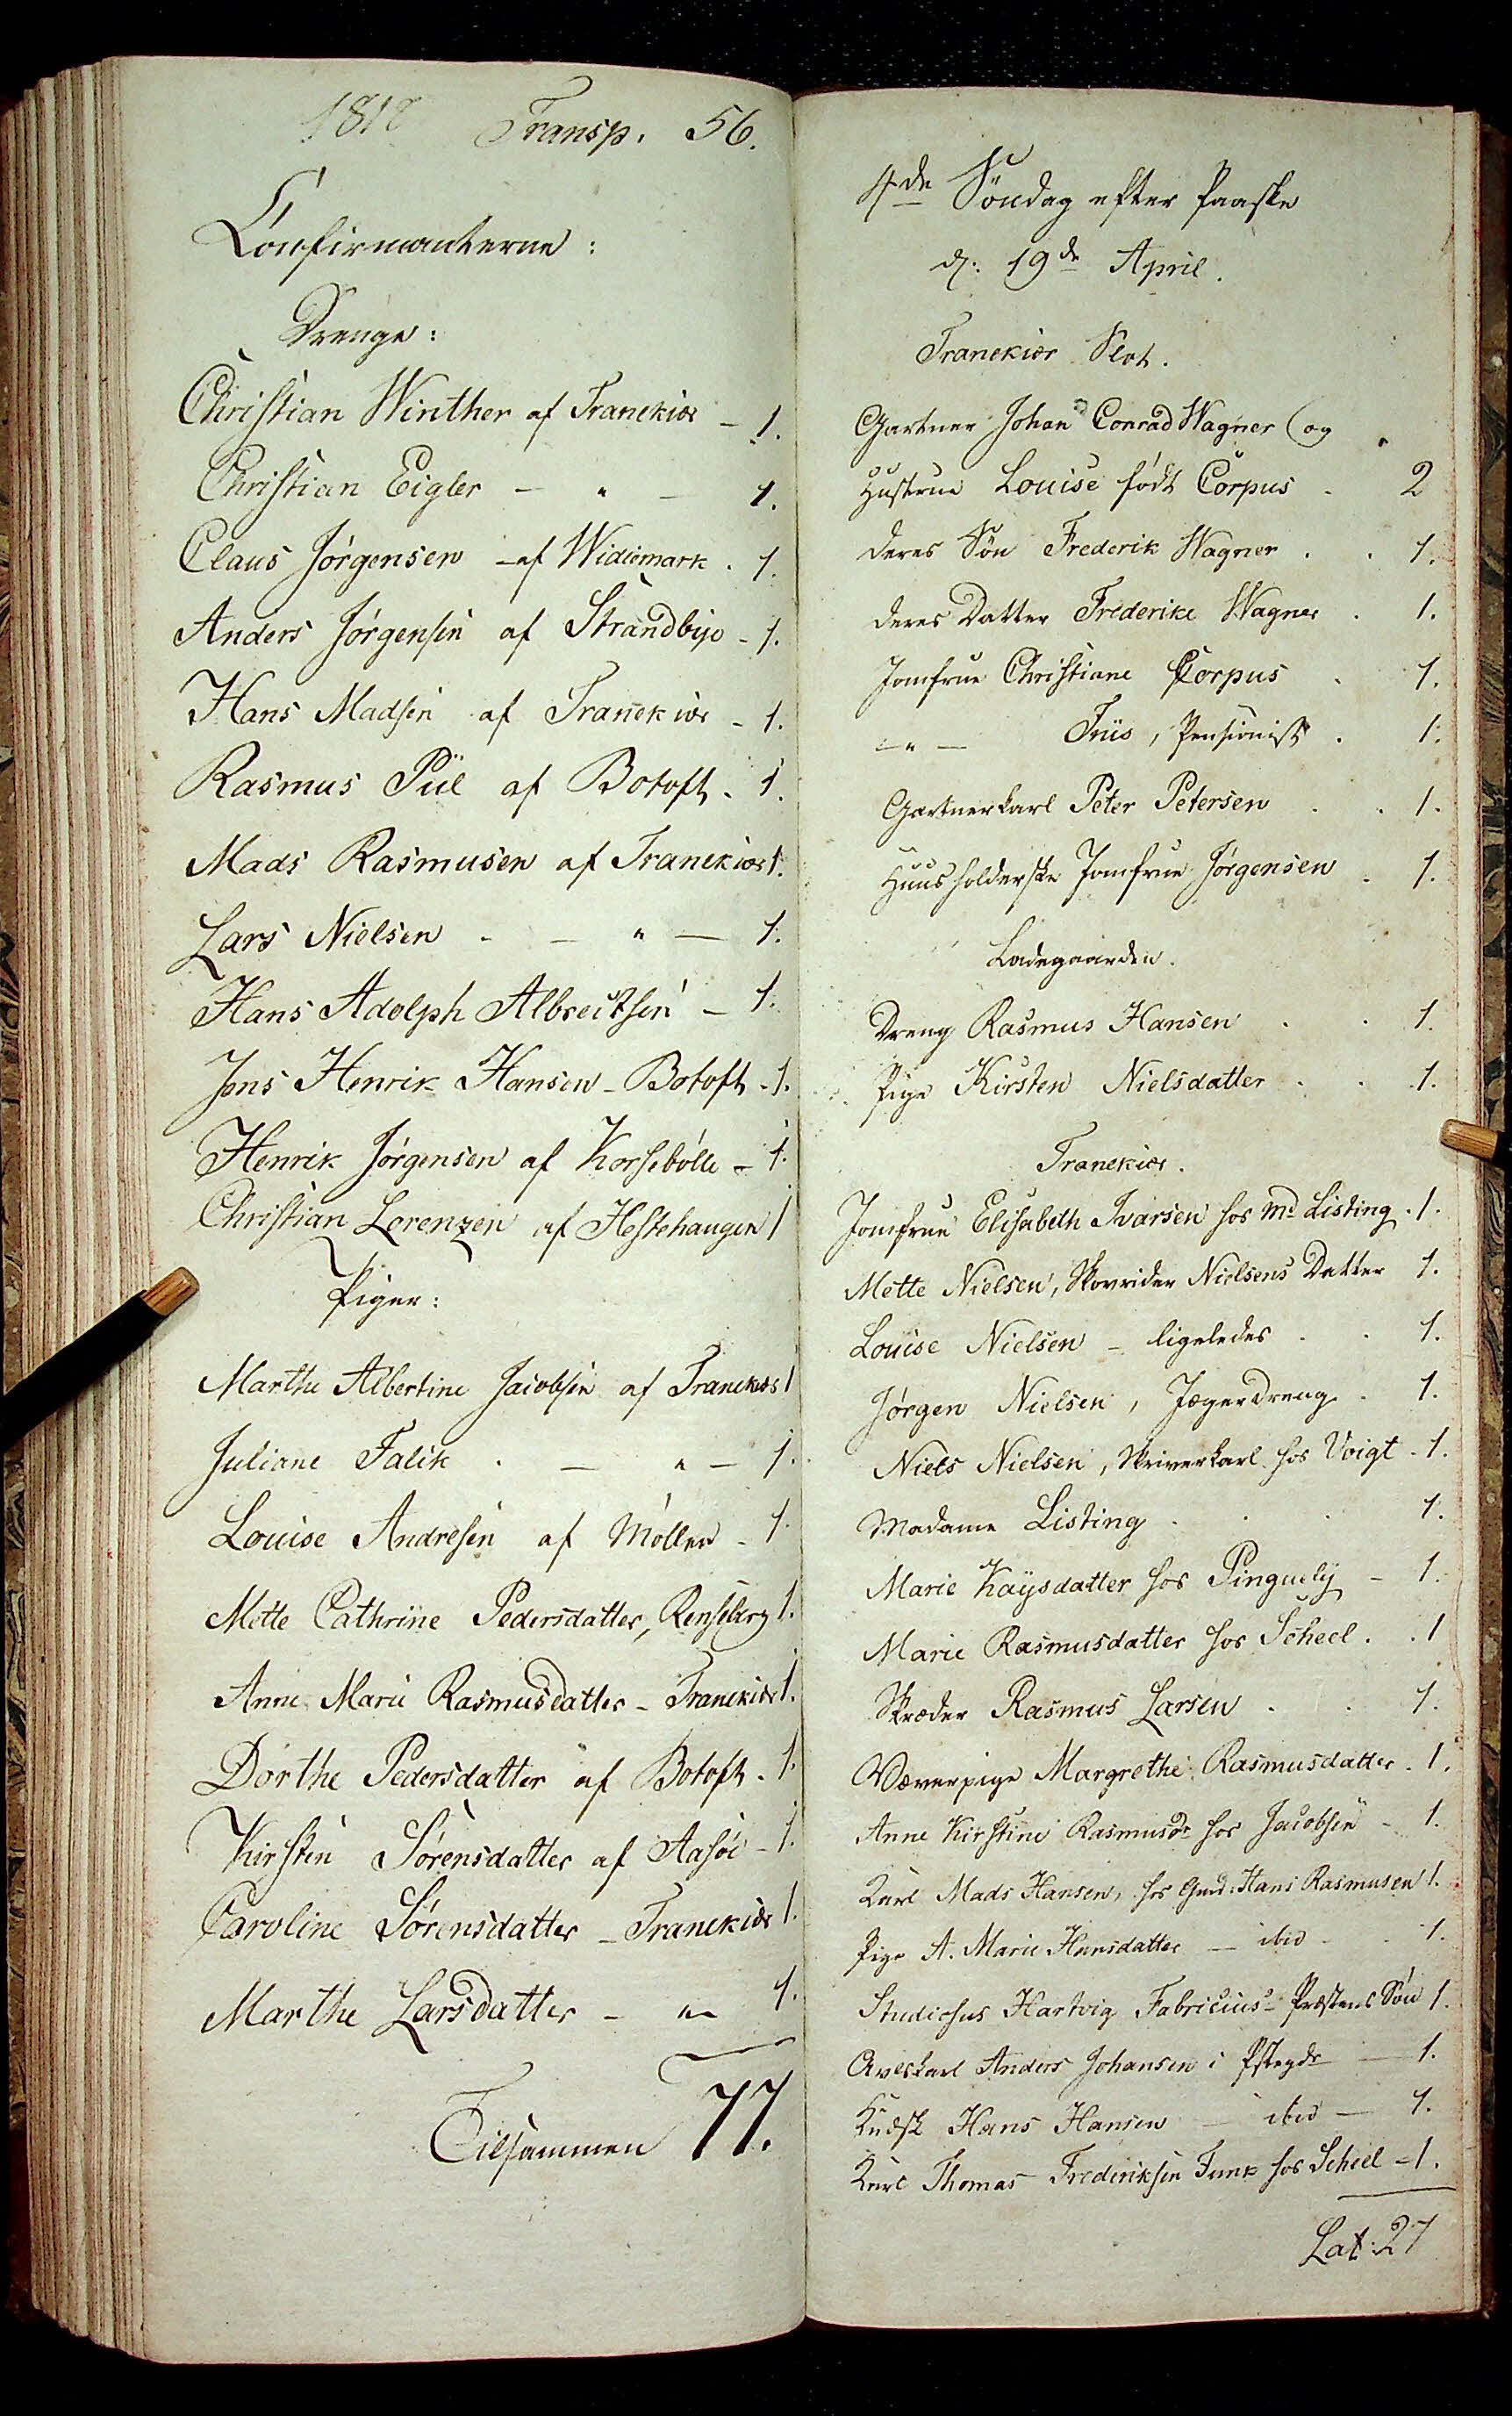1818
Transp. 56.
Confirmanterne:
Drenge:
Christian Wirther af Tranekiær - 1.
Christian Eigler -"- 1.
Claus Jørgensen af Widiemark . 1.
Anders Jørgensen af Strandbye - 1.
Hans Madsen af Tranekiær - 1.
Rasmus Piil af Botoft . 1.
Mads Rasmusen af Tranekiær 1.
Lars Nielsen - -"- 1.
Hans Adolph Albrectsen - 1.
Jens Henrik Hansens - Botoft . 1.
Henrik Jørgensen af Korsebølle - 1.
Christian Sørensen af Hestehaugen 1
Piger:
Marthe Albertine Jacobsen af Tranekiær 1
Juliane Falck . -"- 1.
Louise Andersen af Møllen - 1
Mette Cathrine Pedersdatter, Renseberg 1.
Anne Marie Rasmusdatter - Tranekiær 1.
Dorthe Pedersdatter af Botoft . 1.
Kirsten Sørensdatter af Aasøe - 1.
Caroline Sørensdatter - Tranekiær 1.
Marthe Larsdatter -"- 1.
Tilsammen 77.
4de Søndag efter Paaske
d: 19de April.
Tranekiær Slot.
Gartner, Johan Conrad Wagner og
Hustrue Louise født Corpus . 2
deres Søn Frederik Wagner . . 1.
deres Datter Friderike Wagner . 1.
Jomfrue Christiane Corpus . 1.
-"- Friis Pensionist . 1.
Gartnerkarl Peter Petersen . . 1.
Huusholderse Jomfrue Jørgensen . 1.
Ladegaarden.
Dreng Rasmus Hansen . . 1.
Pige Kirsten Nielsdatter . . . 1.
Tranekiær.
Jomfrue Elisabeth Ivarsen hos Md Listing . 1.
Mette Nielsen, Skovrider Nielsens Datter 1.
Louise Nielsen - ligeledes . . 1.
Jørgen Nielsen, Jægerdreng . 1.
Niels Nielsen, Skriverkarl hos Voigt . 1.
Madame Listing . . . 1.
Marie Kaysdatter hos Pinguely - 1.
Marie Rasmusdatter hos Scheel . . 1
Skræder Rasmus Larsen . . 1.
Væverpige, Margrethe Rasmusdatter . 1.
Anne Kirstine Rasmusdr hos Jacobsen - 1.
Karl Mads Hansen, hos Grd: Hans Rasmusen 1.
Pige A. Marie Hansdatter - ibid . . 1.
Studiosus Hartvig Fabricius - Præstens Søn 1.
Avlskarl Anders Johansen i Pstegdr - 1.
Kudsk Hans Hansen - ibid - 1.
Karl Thomas Frideriksen Funk hos Scheel
Lat: 27
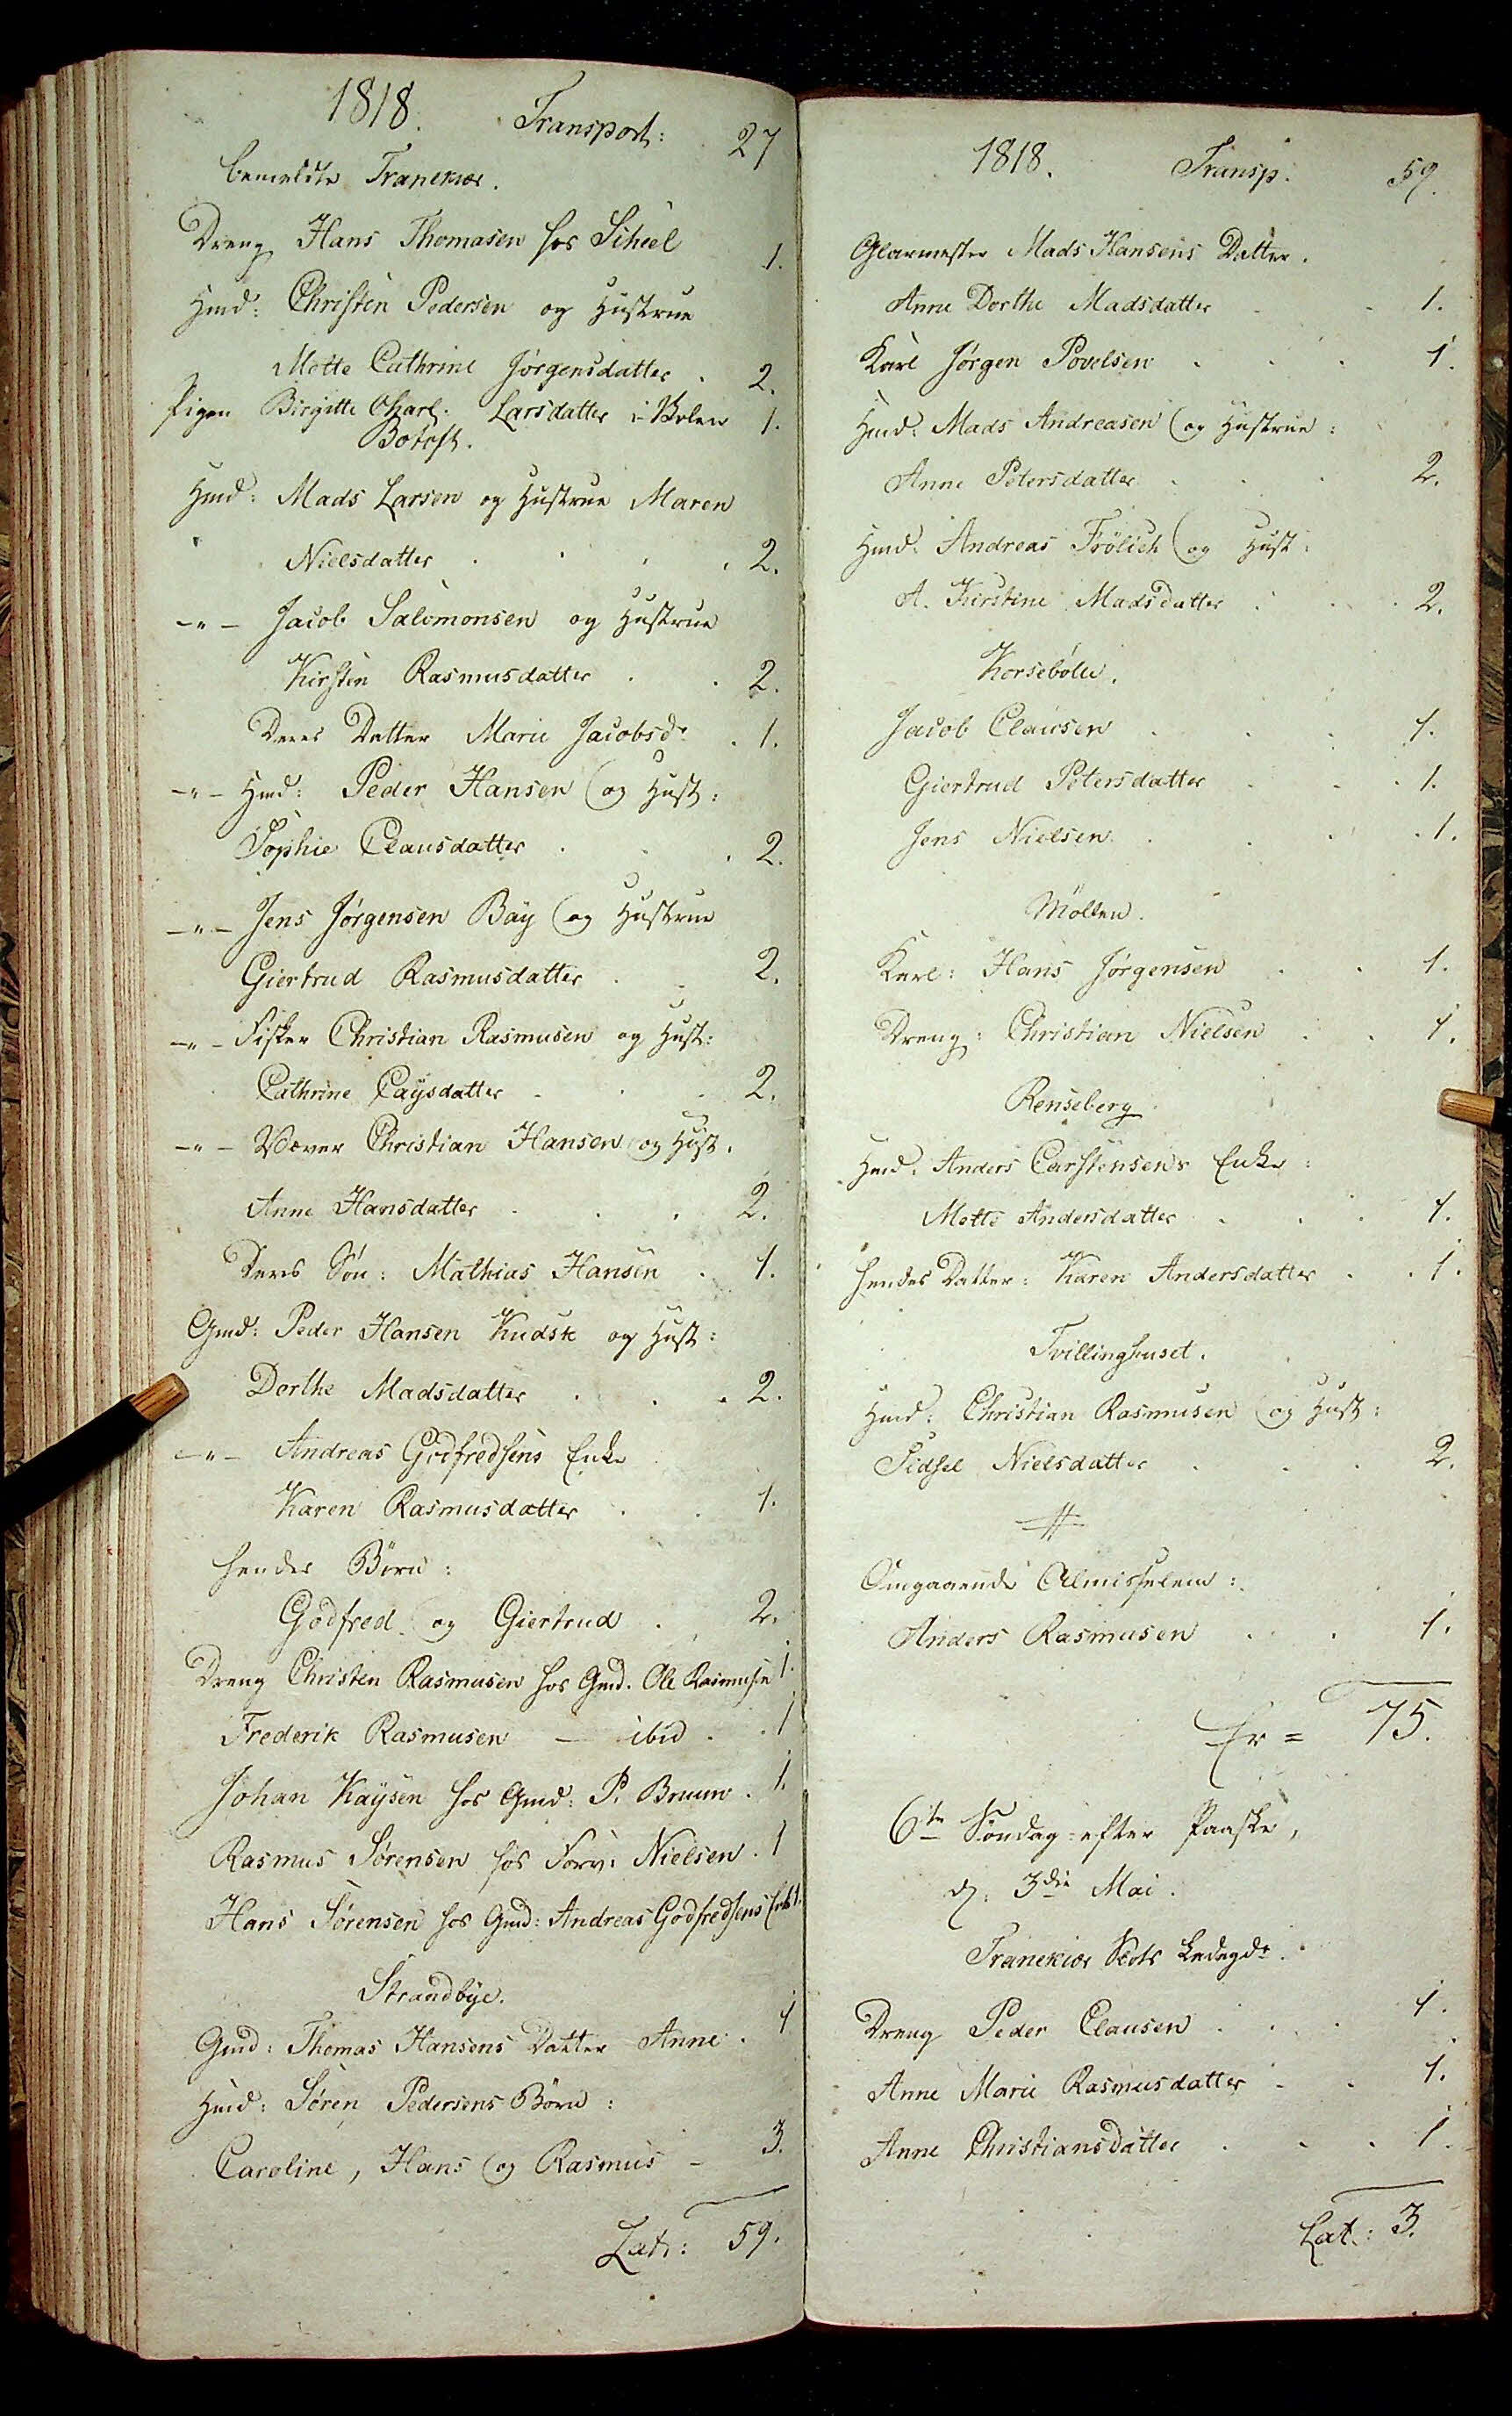1818. . Transport: 27.
bemeldte Tranekiær.
Dreng Hans Thomasen hos Scheel 1.
Hmd: Christen Pedersen og Hustrue
Mette Cathrine Jørgensdatter . 2.
Pigen Birgitte Charl. Larsdatter i Skolen 1.
Botoft.
Hmd: Mads Larsen og Hustrue Maren
Nielsdatter . . . . 2.
-"- Jacob Salomonsen og Hustrue
Kirsten Rasmusdatter . . 2.
Deres Datter Marie Jacobsdr . 1.
-"- Hmd: Peder Hansen og Hust:
Sophie Clausdatter . . . 2.
-"- Jens Jørgensen Bay og Hustrue
Giertrud Rasmusdatter . . 2.
-"- Fisker Christian Rasmusen og Hustr:
Cathrine Caysdatter . . . 2.
-"- Væver Christian Hansen og Hust:
Anne Hansdatter . . . 2.
Deres Søn: Mathias Hansen . 1.
Gmd: Peder Hansen Kudsk og Hust:
Dorthe Madsdatter . . . 2.
-"- Andreas Godfredsens Enke
Karen Rasmusdatter . . 1.
hendes Børn:
Godfred og Giertrud . 2.
Dreng Christen Rasmusen hos Gmd. Ole Rasmusen 1.
Frederik Rasmusen - ibid . .1
Johan Kaysen hos Gmd: P. Bruun . 1
Rasmus Sørensen hos Forv: Nielsen . 1
Hans Sørensen hos Gmd: Andreas Godfredsens Enke 1
Strandbye.
Gmd: Thomas Hansens Datter Anne . 1
Hmd: Søren Pedersens Børn:
Caroline, Hans og Rasmus - 3.
Lat: 59.
1818.
Transp. 59.
Glarmester i Hans Hansens Datter.
Anne Dorthe Madsdatter . . 1.
Karl Jørgen Povelsen . . . 1
Hmd: Mads Andreasen og Hustrue:
Anne Petersdatter . . . 2.
Hmd: Andreas Frølich og Hust:
A. Kirstine Madsdatter . . . 2.
Korsebølle.
Jacob Clausen . . . 1.
Giertrud Pedersdatter . . . 1.
Jens Nielsen . . . . 1.
Møllen.
Karl: Hans Jørgensen . . 1.
Dreng: Christian Nielsen . .1.
Renseberg
Hmd: Anders Carstensens Enke
Mette Andersdatter . . . 1.
hendes Datter: Karen Andersdatter . . 1.
Tvillinghuset.
Hmd: Christian Rasmusen og Hust:
Sidsel Nielsdatter . . . 2.
Omgaaende Almisselem:
Anders Rasmusen . . . 1.
Er = 75.
6te Søndag efter Paaske,
d: 3die Mai.
Tranekiær Slots Ladegdr.
Dreng Peder Clausen . . . 1.
Anne Marie Rasmusdatter . . 1.
Anne Christiansdatter . . . 1.
Lat: 3.